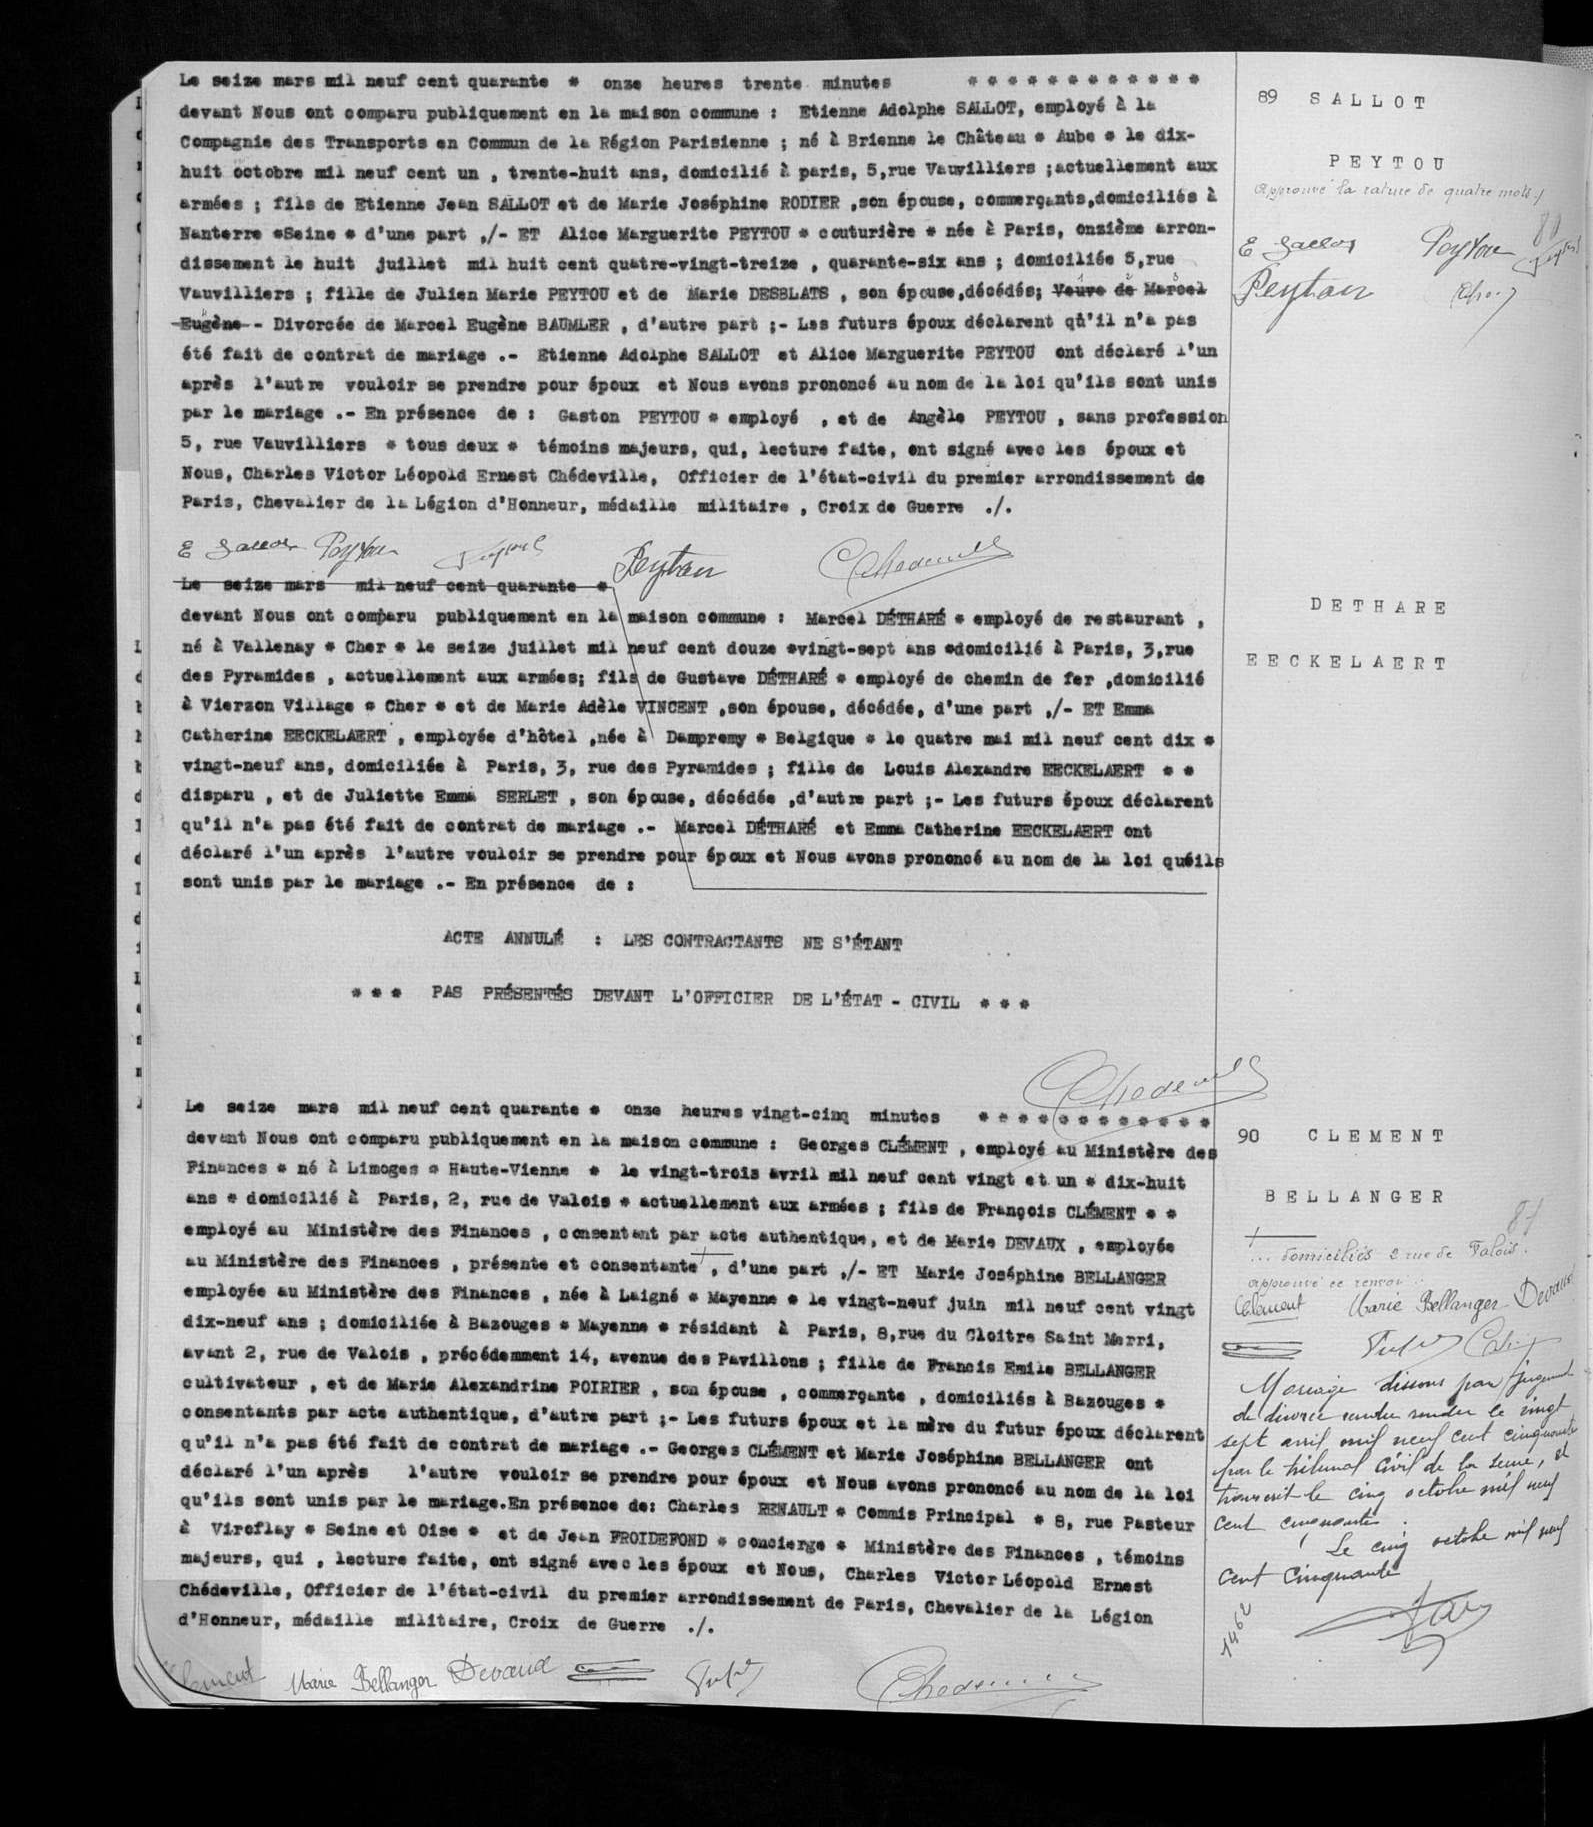Le seize mars mil neuf cent quarante * onze heures trentes minutes ****
devant Nous ont comparu publiquement en la maison commune: Etienne Adolphe SALLOT, employé à la
Compagnie des Transports en Commun de la Région Parisienne; né à Brienne le Château * Aube * le dix-
huit octobre mil neuf cent un, trente-huit ans, domicilié à paris, 5, rue Vauvilliers; actuellement aux
armées; fils de Etienne Jean SALLOT et de Marie Joséphine RODIER, son épouse, commerçants, domiciliés à
Nanterre * Seine * d'une part ,/-ET Alice Marguerite PEYTOU * couturière * née à Paris, onzième arron-
dissement le huit juillet mil huit cent quatre-vingt-treize, quarante-six ans; domiciliés 5, rue
Vauvilliers; fille de Julien Marie PEYTOU et de Marie DESBLATS, son épouse, décédés; Veuve de Marcel
Eugène-Divorcée de Marcel Eugène BAUMLER, d'autre part ;-Les futurs époux déclarent qu'il n'a pas
été fait de contrat de mariage .-Etienne Adolphe SALLOT et Alix Marguerite PEYTOU ont déclaré l'un
après l'autre vouloir se prendre pour époux et Nous avons prononcé au nom de la loi qu'ils sont unis
par le mariage .-En présence de; Gaston PEYTOU * employé, et de Angèle PEYTOU, sans profession
5, rue Vauvilliers * tous deux * témoins majeurs, qui, lecture faite, ont signé avec les époux et
Nous, Charles Victor Léopold Ernest Chédeville, Officier de l'état-civil du premier arrondissement de
Paris, Chevalier de la Légion d'Honneur, médaille militaire, Croix de Guerre ./.
Le seize mars mil neuf cent quarante *
devant Nous ont comparu publiquement en la maison commune; Marcel DETHARE * employé de restaurant,
né à Vallenay * Cher * le seize juillet mil neuf cent doux * vingt-sept ans * domicilié à Paris, 3, rue
des Pyramides, actuellement aux armées; fils de Gustave DETHARE * employé de chemin de fer, domicilié
à Vierzon Village * Cher * et de Marie Adèle VINCENT, son épouse, décédée, d'une part ,/-ET Emma
Chatherine BECKELAERT, employée d'hôtel, née à Dampremy * Belgique * le quatre mai mil neuf cent dix *
vingt-neuf ans, domiciliée à Paris, 3, rue des Pyramides; fille de Louis Alexandre EECKELAERT **
disparu, et de Juliette Emma SERLET, son épouse, décédée, d'autre part ;-Les futurs époux déclarent
qu'il n'a pas été fait de contrat de mariage .-Marchel DETHARE et Emma Catherine EECKELAERT ont
déclaré l'un après l'autre vouloir se prendre pour époux et Nous avons prononcé au nom de la loi qu'ils
sont unis par le mariage .-En présence de
ACTE ANNULE: LES CONTRACTANTS NE S'ETANT
*** PAS PRESENTES DEVANT L'OFFICIER DE L'ETAT-CIVIL ***
Le seize mars mil neuf cent quarante * onze heures vingt-cinq minutes ****
devant Nous ont comparu publiquement en la maison commune: Georges CLEMENT, employé au Ministère des
Finances * né à Limoges * Haute-Vienne * le vingt-trois avril mil neuf cent vingt et un * dix-huit
ans * domicilié à Paris, 2, rue des Valois * actuellement aux armées; fils de François CLEMENT **
employé au Ministère des Finances, consentant par acte authentique, et de Marie DEVAUX, employée
au Ministère des Finances, présente et consentante, d'une part ,/-ET Marie Joséphine BELLANGER
employée au Ministère des Finances, née à Laigné * Mayenne * le vingt-neuf juin mil neuf cent vingt
dix-neuf ans; domiciliée à Bazouges * Mayenne * résidant à Paris, 8, rue du Cloitre Saint Merri,
avant 2, rue de Valois, précédemment 14, avenue des Pavillons; fille de Francis Emile BELLANGER
cultivateur, et de Marie Alexandrine POIRIER, son épouse, commerçante, domiciliés à Bazouges *
consentants par acte authentique, d'autre part ;-Les futurs époux et la mère du futur époux déclarent
qu'il n'a pas été fait de contrat de mariage .-Georges CLEMENT et Marie Joséphine BELLANGER ont
déclaré l'un après l'autre vouloir se prendre pour époux et Nous avons prononcé au nom de la loi
qu'ils sont unis par le mariage. En présence de: Charles RENAULT * Commis Principal * 8, rue Pasteur
à Viroflay * Seine et Oise * et de Jean FROIDEFOND * concierge * Ministère des Finances, témoins
majeurs, qui, lecture faite, ont signé avec les époux et Nous, Charles Victor Léopold Ernest
Chédeville, Officier de l'état-civil du premier arrondissement de Paris. Chevalier de la Légion
d'Honneur, médaille militaire, Croix de Guerre ./.
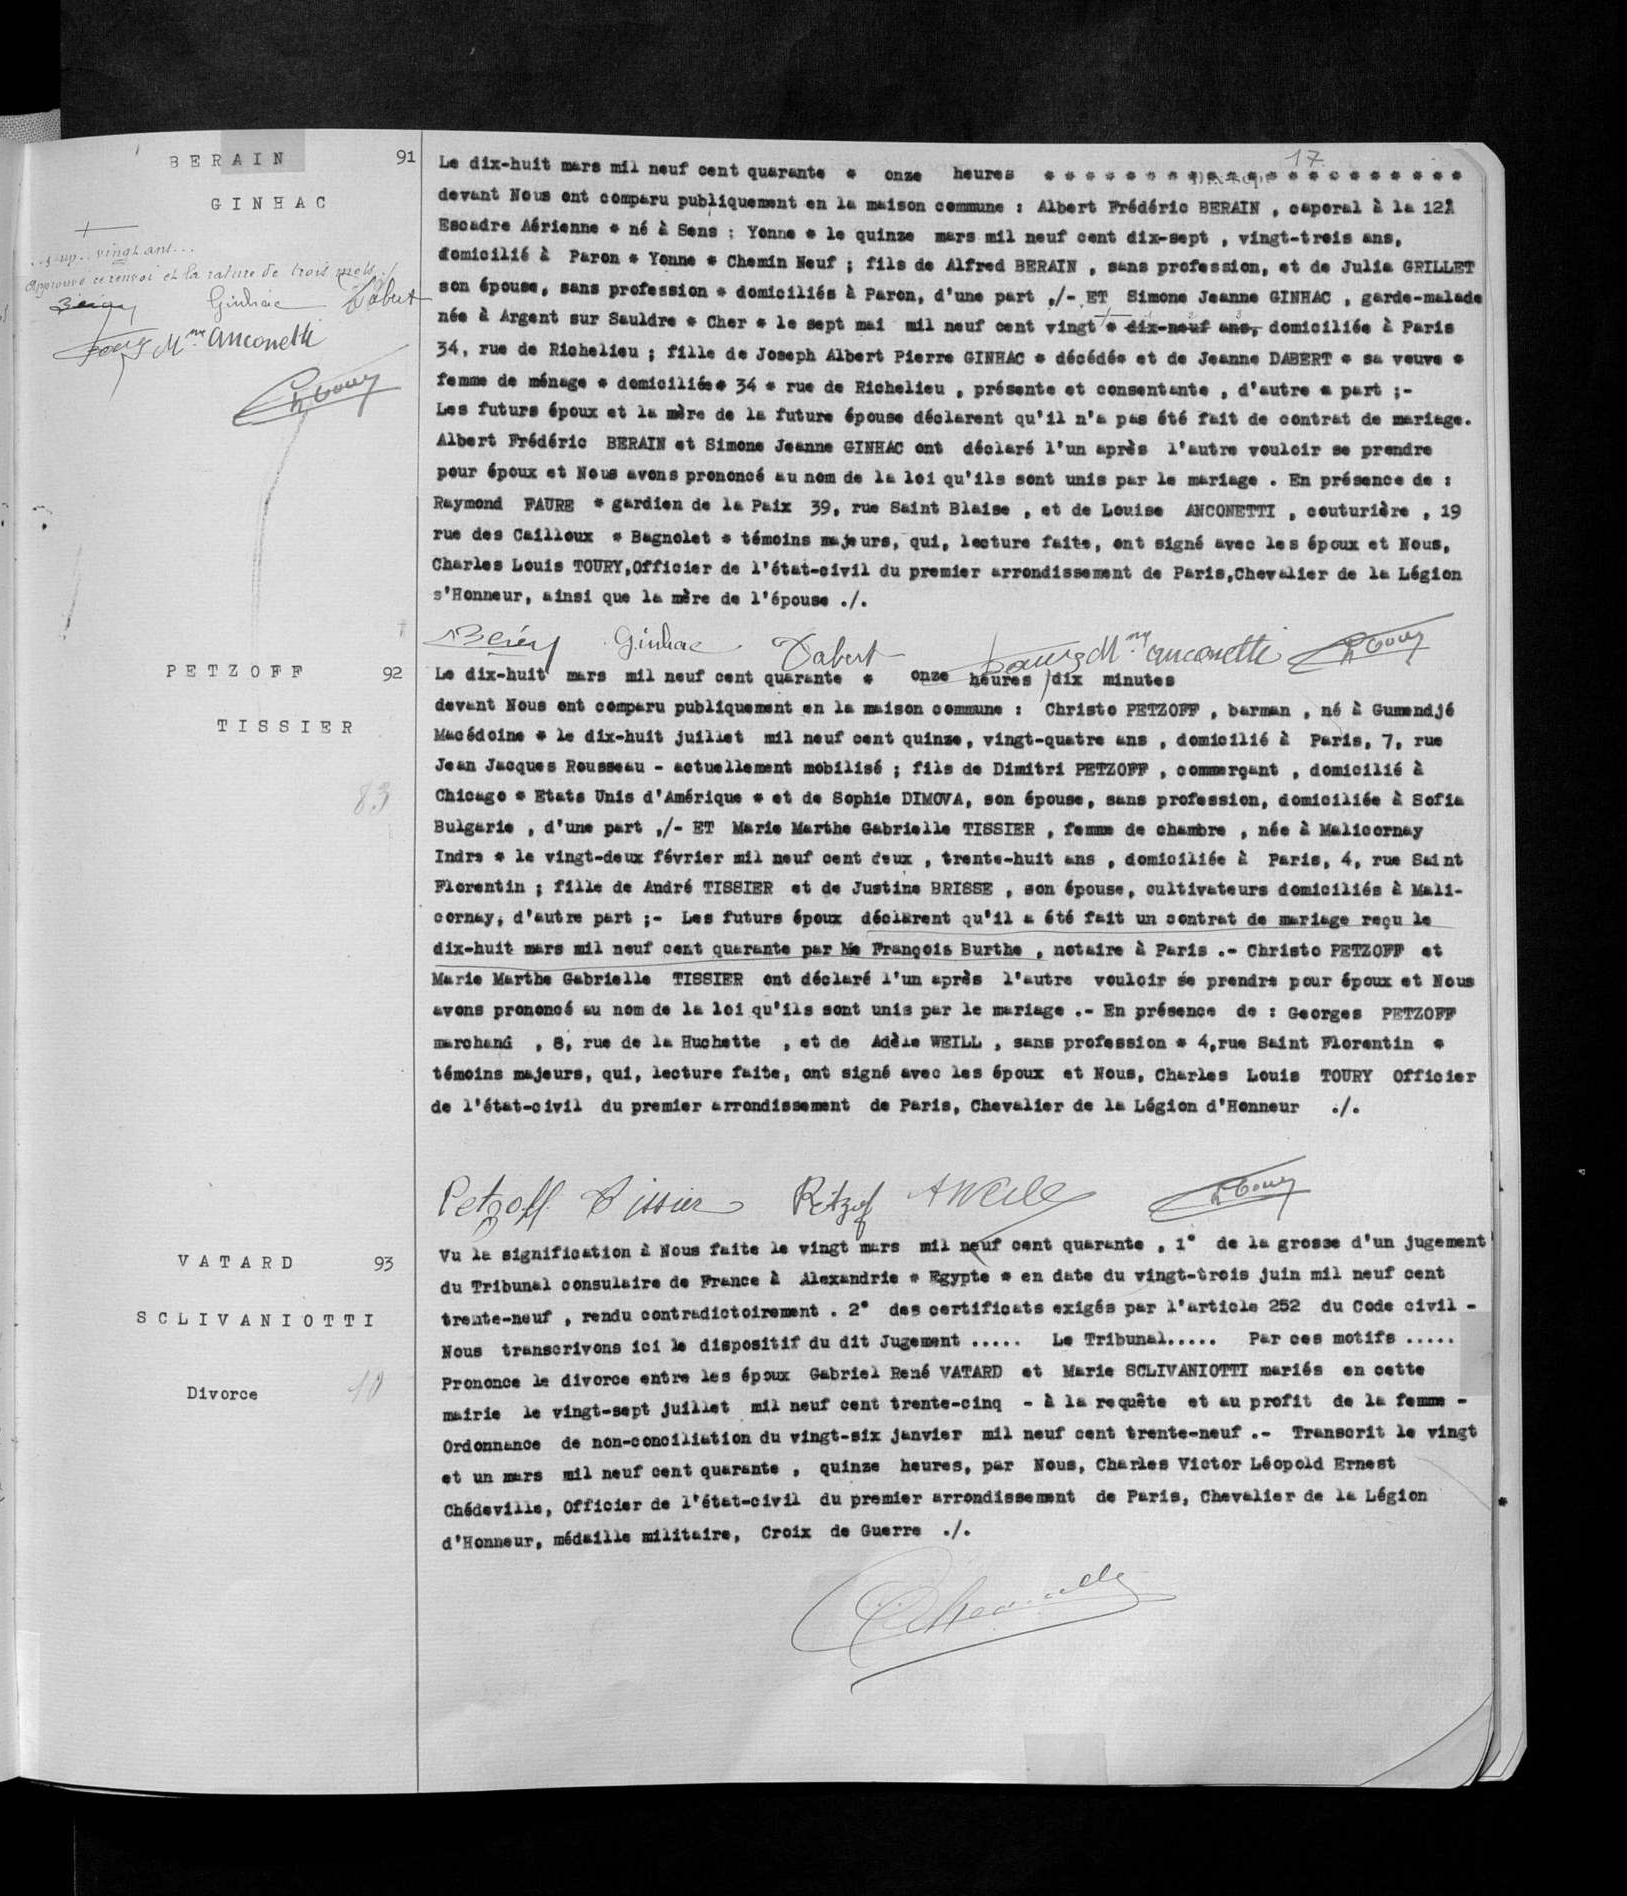Le dix-huit mars mil neuf cent quarante * onze heures ****
devant Nous ont comparu publiquement en la maison commune: Albert Frédéric BERAIN, caporal à la 12A°
Escadre Aérienne * né à Sens: Yonne * le quinze mars mil neuf cent dix-sept, vingt-trois ans,
domicilié à Paron * Yonne * Chemin Neuf; fils de Alfred BERAIN, sans profession, et de Julia GRILLET
son épouse, sans profession * domiciliés à Paron, d'une part ,/-ET Simone Jeanne GINHAC, garde-malade
née à Argent sur Sauldre * Cher * le sept mai mil neuf cent vingt * dix-neuf ans, domiciliée à Paris
34, rue de Richelieu; fille de Joseph Albert Pierre GINHAC * décédé * et de Jeanne DABERT * sa veuve *
femme de ménage * domiciliée * 34 * rue de Richelieu, présente et consentante, d'autre * part;-
Les futurs époux et la mère de la future épouse déclarent qu'il n'a pas été fait de contrat de mariage.
Albert Frédéric BERAIN et Simone Jeanne GINHAC ont déclaré l'un après l'autre vouloir se prendre
pour époux et Nous avons prononcé aux nom de la loi qu'ils sont unis par le mariage. En présence de:
Raymond FAURE * gardien de la Paix 39, rue Saint Blaise, et de Louise ANCONETTI, couturière, 19
rue des Cailloux * Bagnolet * témoins majeurs, qui, lecture faite, ont signé àavec les époux et Nous,
Charles Louis TOURY, Officier de l'état-civil du premier arrondissement de Paris, Chevalier de la Légion
d'Honneur, ainsi que la mère de l'épouse ./.
Le dix-huit mars mil neuf cent quarante * onze heures dix minutes
devant Nous ont comparu publiquement en la maison commune: Christo PETZOFF, barman, né à Gumendjé
Macédoine * le dix-huit juillet mil neuf cent quinze, vingt-quatre ans, domicilié à Paris, 7, rue
Jean Jacques Rousseau-actuellement mobilisé; fils de Dimitri PETZOFF, commerçant, domicilié à
Chicago * Etats Unis d'Amérique * et de Sophie DIMOVA, son épouse, sans profession, domiciliée à Sofia
Bulgarie, d'une part ,/-ET Marie Marthe Gabrielle TISSIER, femme de chambre, née à Malicornay
Indre * le vingt-deux février mil neuf cent deux, trente-huit ans, domiciliée à Paris, 4, rue Saint
Florentin; fille de André TISSIER et de Justine BRISSE, son épouse, cultivateurs domiciliés à Mali-
cornay, d'autre part ;-Les futurs époux déclarent qu'il a été fait un contrat de mariage reçu le
dix-huit mars mil neuf cent quarante par Me François Burthe, notaire à Paris .-Christo PETZOFF et
Marie Marthe Gabrielle TISSIER ont déclaré l'un après l'autre vouloir se prendre pour époux et Nous
avons prononcé au nom de la loi qu'ils ont unis par le mariage .-En présence de: Georges PETZOFF
marchand, 8, rue de la Huchette, et de Adèle WEILL, sans profession * 4, rue Saint Florentin *
témoins majeurs, qui, lecture faite, ont signé avec les époux et Nous, Charles Louis TOURY Officier
de l'état-civil du premier arrondissement de Paris, Chevalier de la Légion d'Honneur ./.
Vu la signification à Nous faite le vingt mars mil neuf cent quarante, 1° de la grosse d'une jugement
du Tribunal consulaire de France à Alexandrie * Egypte * en date du vingt-trois juin mil neuf cent
trente-neuf, rendu contradictoirement. 2° des certificats exigés par l'article 252 du Code civil-
Nous transcrivons ici le dispositif du dit Jugement.... Le Tribunal.... Par ce motifs...
Prononce le divorce entre les époux Gabriel René VATARD et Marie SCLIVANIOTTI mariés en cette
mairie le vingt-sept juillet mil neuf cent trente-cinq-à la requête et au profit de la femme-
Ordonnance de non-conciliation du vingt-six janvier mil neuf cent trente-neuf .-Transcrit le vingt
et un mars mil neuf cent quarante, quinze heures, par Nous, Charles Victor Léopold Ernest
Chédeville, Officier de l'état-civil du premier arrondissement de Paris, Chevalier de la Légion
d'Honneur, médaille militaire, Croix de Guerre ./.